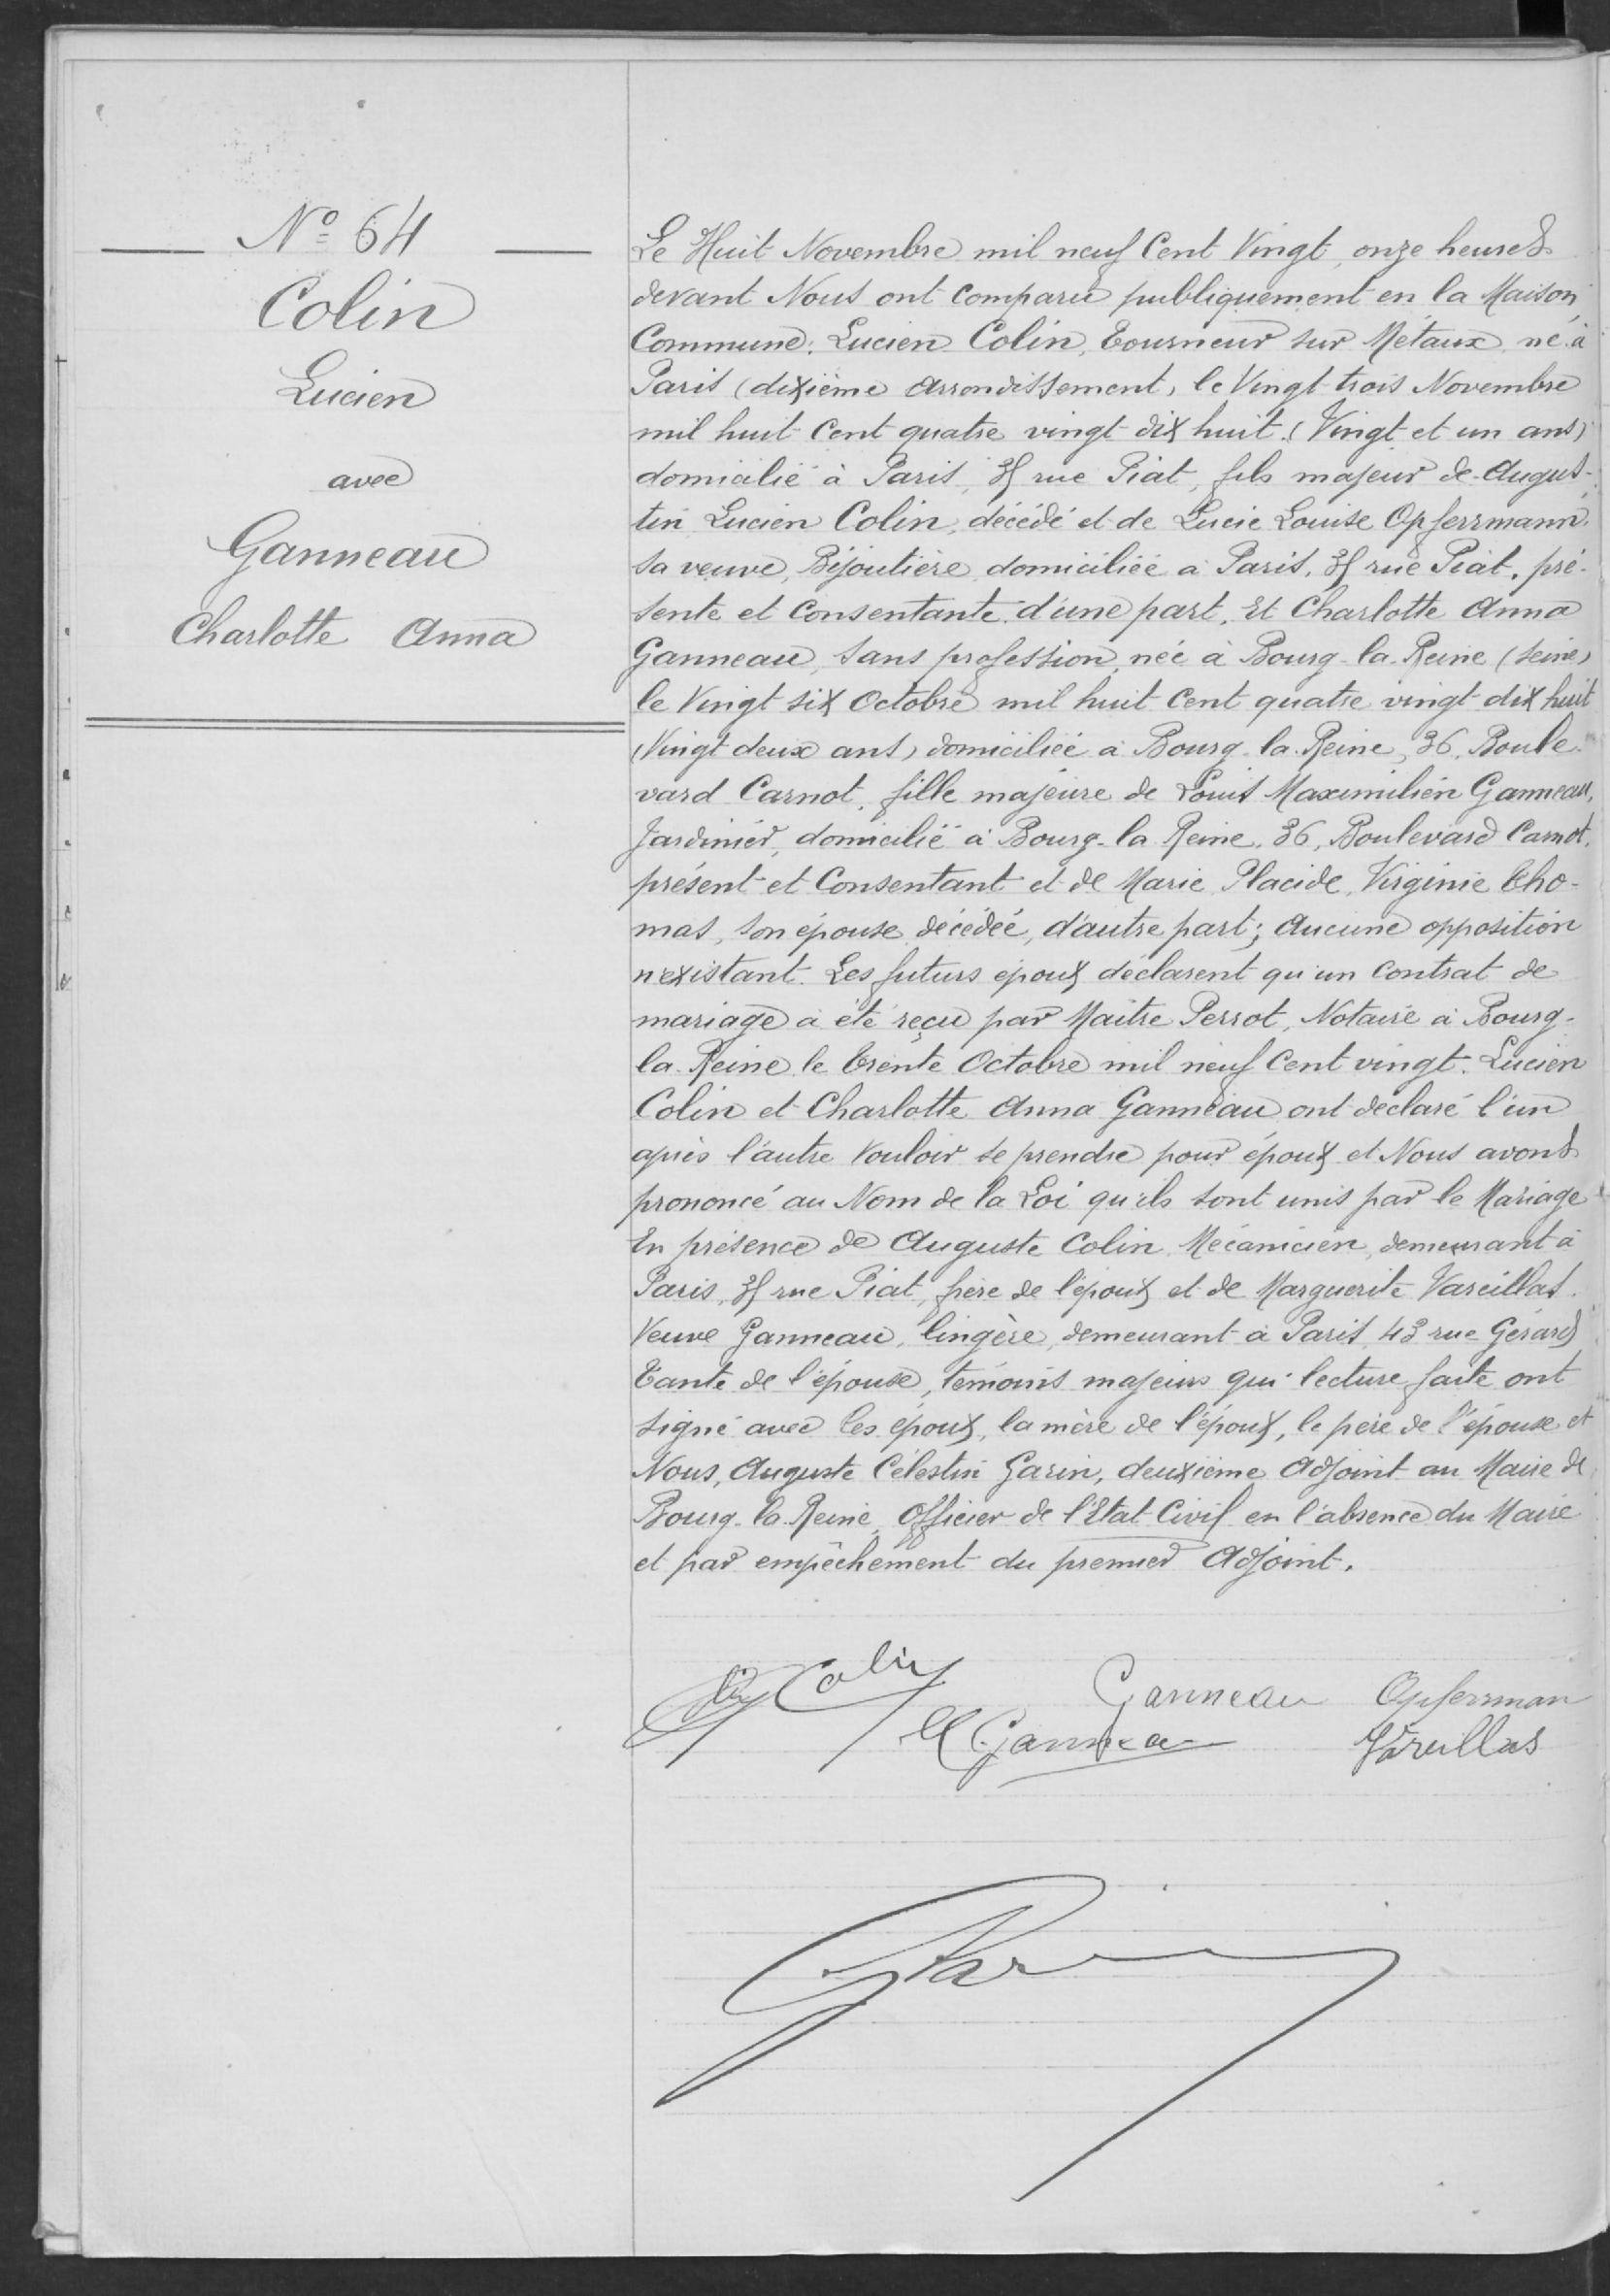N°64
Colin
Lucien
avec
Ganneau
Charlotte Anna
Le huit Novembre mil neuf cent vingt, onze heures
devant Nous ont comparu publiquement en la Maison
Commune: Lucien Colin Tourneur sur Métaux, né à
Paris (dixième arrondissement) le vingt trois Novembre
mil huit cent quatre vingt-dix huit (vingt et un ans)
domicilié à Paris, 38 rue Piat, fils majeur de Augus-
tin Lucien Colin, décédé et de Lucie Louise Opfermam,
sa veuve, bijoutière domiciliée à Paris, 38 rue Piat, pré-
sente et consentante d'une part, Et Charlotte Anna
Ganneau, sans profession, née à Bourg-la-Reine (Seine)
le vingt six octobre mil huit cent quatre vingt-dix-huit
(vingt-deux ans) domiciliée à Bourg-la-Reine, 36 Boule
vard Carnot, fille majeure de Louis Maximilien Gammeau,
jardinier, domicilié à Bourg-la-Riene, 36, Boulevard Carnot,
présent et consentant et de Marie Placide, Virginie Tho-
mas, son épouse décédée, d'autre part; aucune opposition
n'existant. Les futurs époux déclarent qu'un contrat de
mariage a été reçu par Maitre Perrot, Notaire à Bourg-
la-Reine le trente octobre mil neuf cent vingt. Lucien
Colin et Charlotte Anna Ganneau ont déclaré l'un
après l'autre vouloir se prendre pour époux et Nous avons
prononcé au Nom de la Loi qu'ils sont unis par le Mariage
En présence de Auguste Colin Mécanicien demeurant à
Paris, 38 rue Piat frère de l'époux et de Marguerite Vareillas.
Veuve Ganneau, lingère, demeurant à Paris, 43 rue Gérard
tante de l'épouse, témoins majeurs qui lecture faite ont
signé avec les époux, la mère de l'époux, le père de l'épouse et
Nous, Auguste Célestin Garin, deuxième adjoint au Maire 4
Bourg-la-Reine, Officier de l'Etat Civil en l'absence du Marie
et par empêchement du premier adjoint.
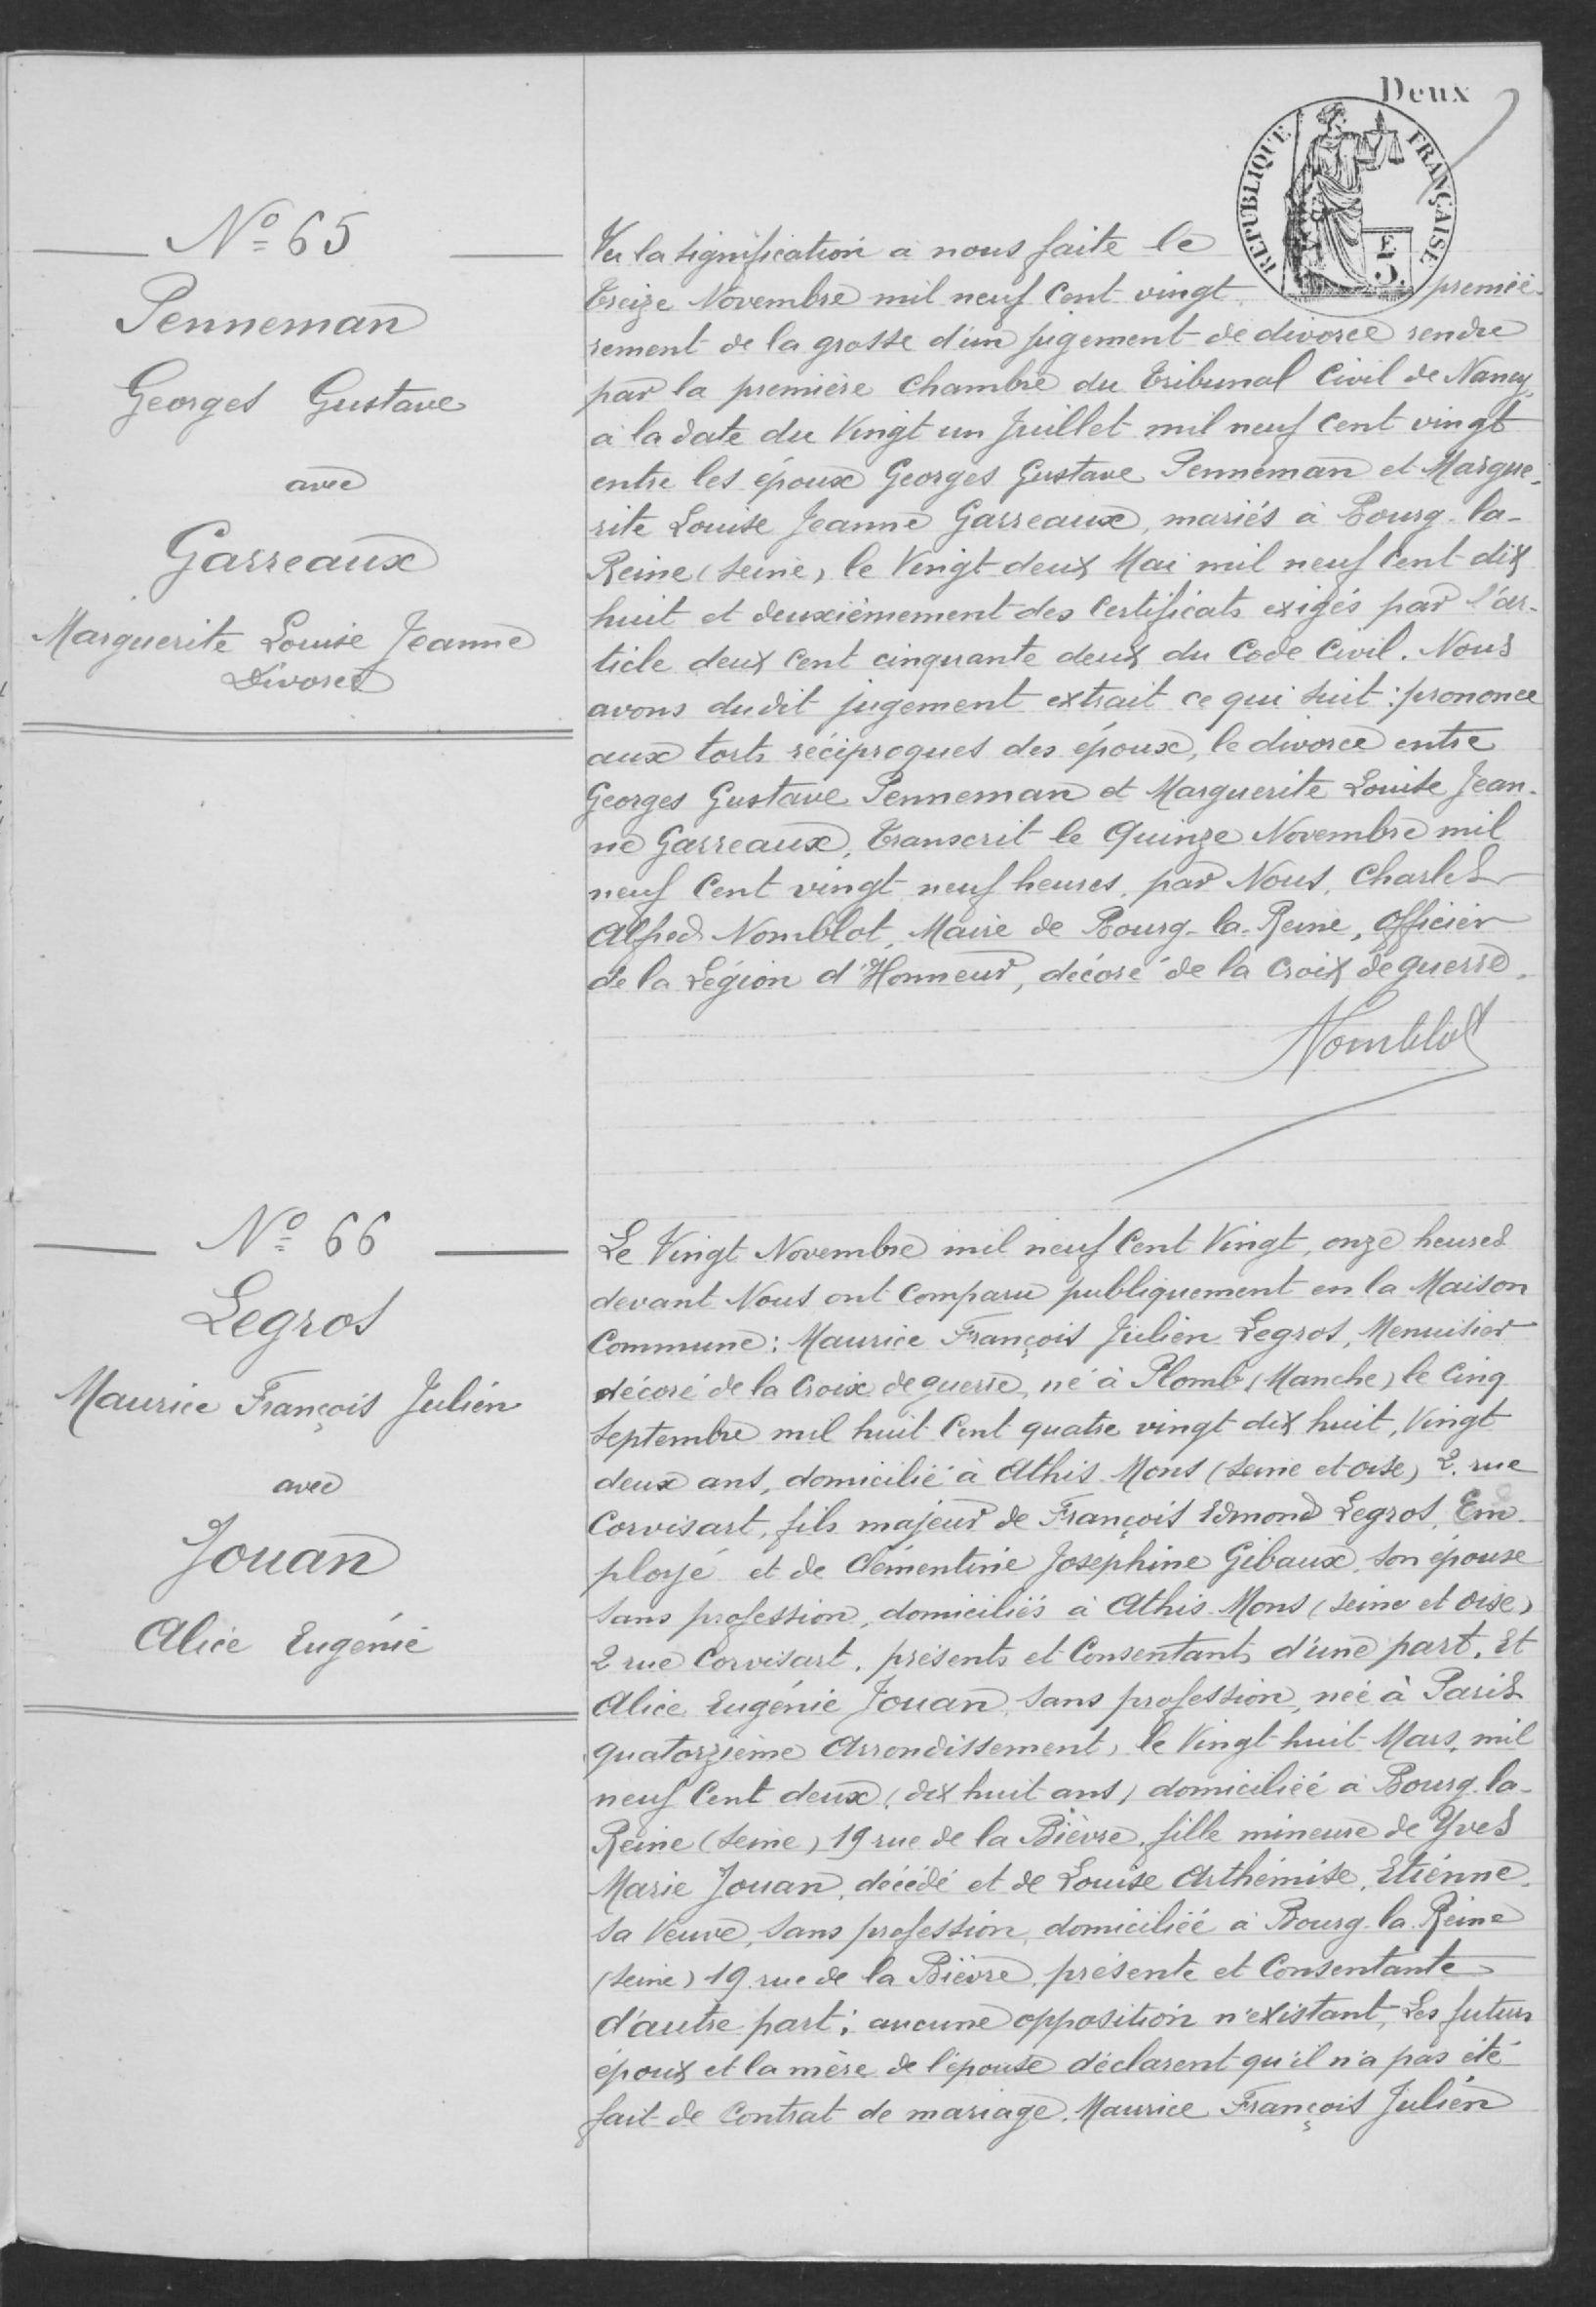N°65
Penneman
Georges Gustave
avec
Gasseaux
Marguerite Louise Jeanne
Livoser
Vu la signification a nous faite le
treize Novembre mil neuf cent vingt première
rement de la grasse d'un jugement de divorce rendu
par la première chambre du Tribunal Civil de Nancy,
à la date du vingt un juillet mil neuf cent vingt
entre les époux Georges Gustave Penneman et Margue-
rite Louise Jeanne Gasseaux, mariés à Bourg-la
Reine (Seine) le vingt-deux Mai mil neuf cent-dix
huit et deuxièmement des certificats exigés par l'ar-
ticle deux cent cinquante deux du code civil. Nous
avons dudit jugement extrait ce qui suit: prononce
aux torts réciproques des époux, le divorce entre
Georges Gustave Penneman et MArguerite Louise Jean-
ne Gasseaux, transcrit le quinze Novembre mil
neuf cent vingt neuf heures, par Nous, Charles
Alfred Nomblot, Mairie de Bourg-la-Reine, Officier
de la Légion d'Honneur, décoré de la Croix de guerre.
N°: 66
Legros
Maurice François Julien
avec
Jouan
Alice Eugénie
Le Vingt Novembre mil neuf cent vingt, onze heures
devant Nous ont comparu publiquement en la Maison
Commue: Maurice François Julien Legros, Menuisier
décoré de la Croix de guerre, né à Plomb (Manche) le cinq
septembre mil huit cent quatre vingt dix huit, vingt
deux ans, domicilié à Athis Mons (Seine et Oise) 2 rue
Corvisart, fils majeur de François Edmond Legros, em
ployé et de Clémentine Joséphine Gibaux son épouse
sans profession, domiciliés à Athis Mons (Seine et Oise)
2 rue Corvisart, présents et consentants d'une part, et
Alice Eugénie Jouan sans profession, née à Paris
quatorzième arrondissement, le vingt huit Mars mil
neuf cent deux (dix huit ans) domicilié à Bourg-la-
Reine (Seine) 19 rue de la Bièvre, fille mineure de Yves
Marie Jouan, décédé et de Louise Arthémise, Etienne
sa veuve, sans profession, domiciliée à Bourg-la-Reine
(Seine) 19 rue de la Bièvre présente et consentante
d'autre part: aucune opposition n'existant, Les futurs
époux et la mère de l'épouse déclarent qu'il n'a pas été
fait de contrat de mariage. Maurice François Julien